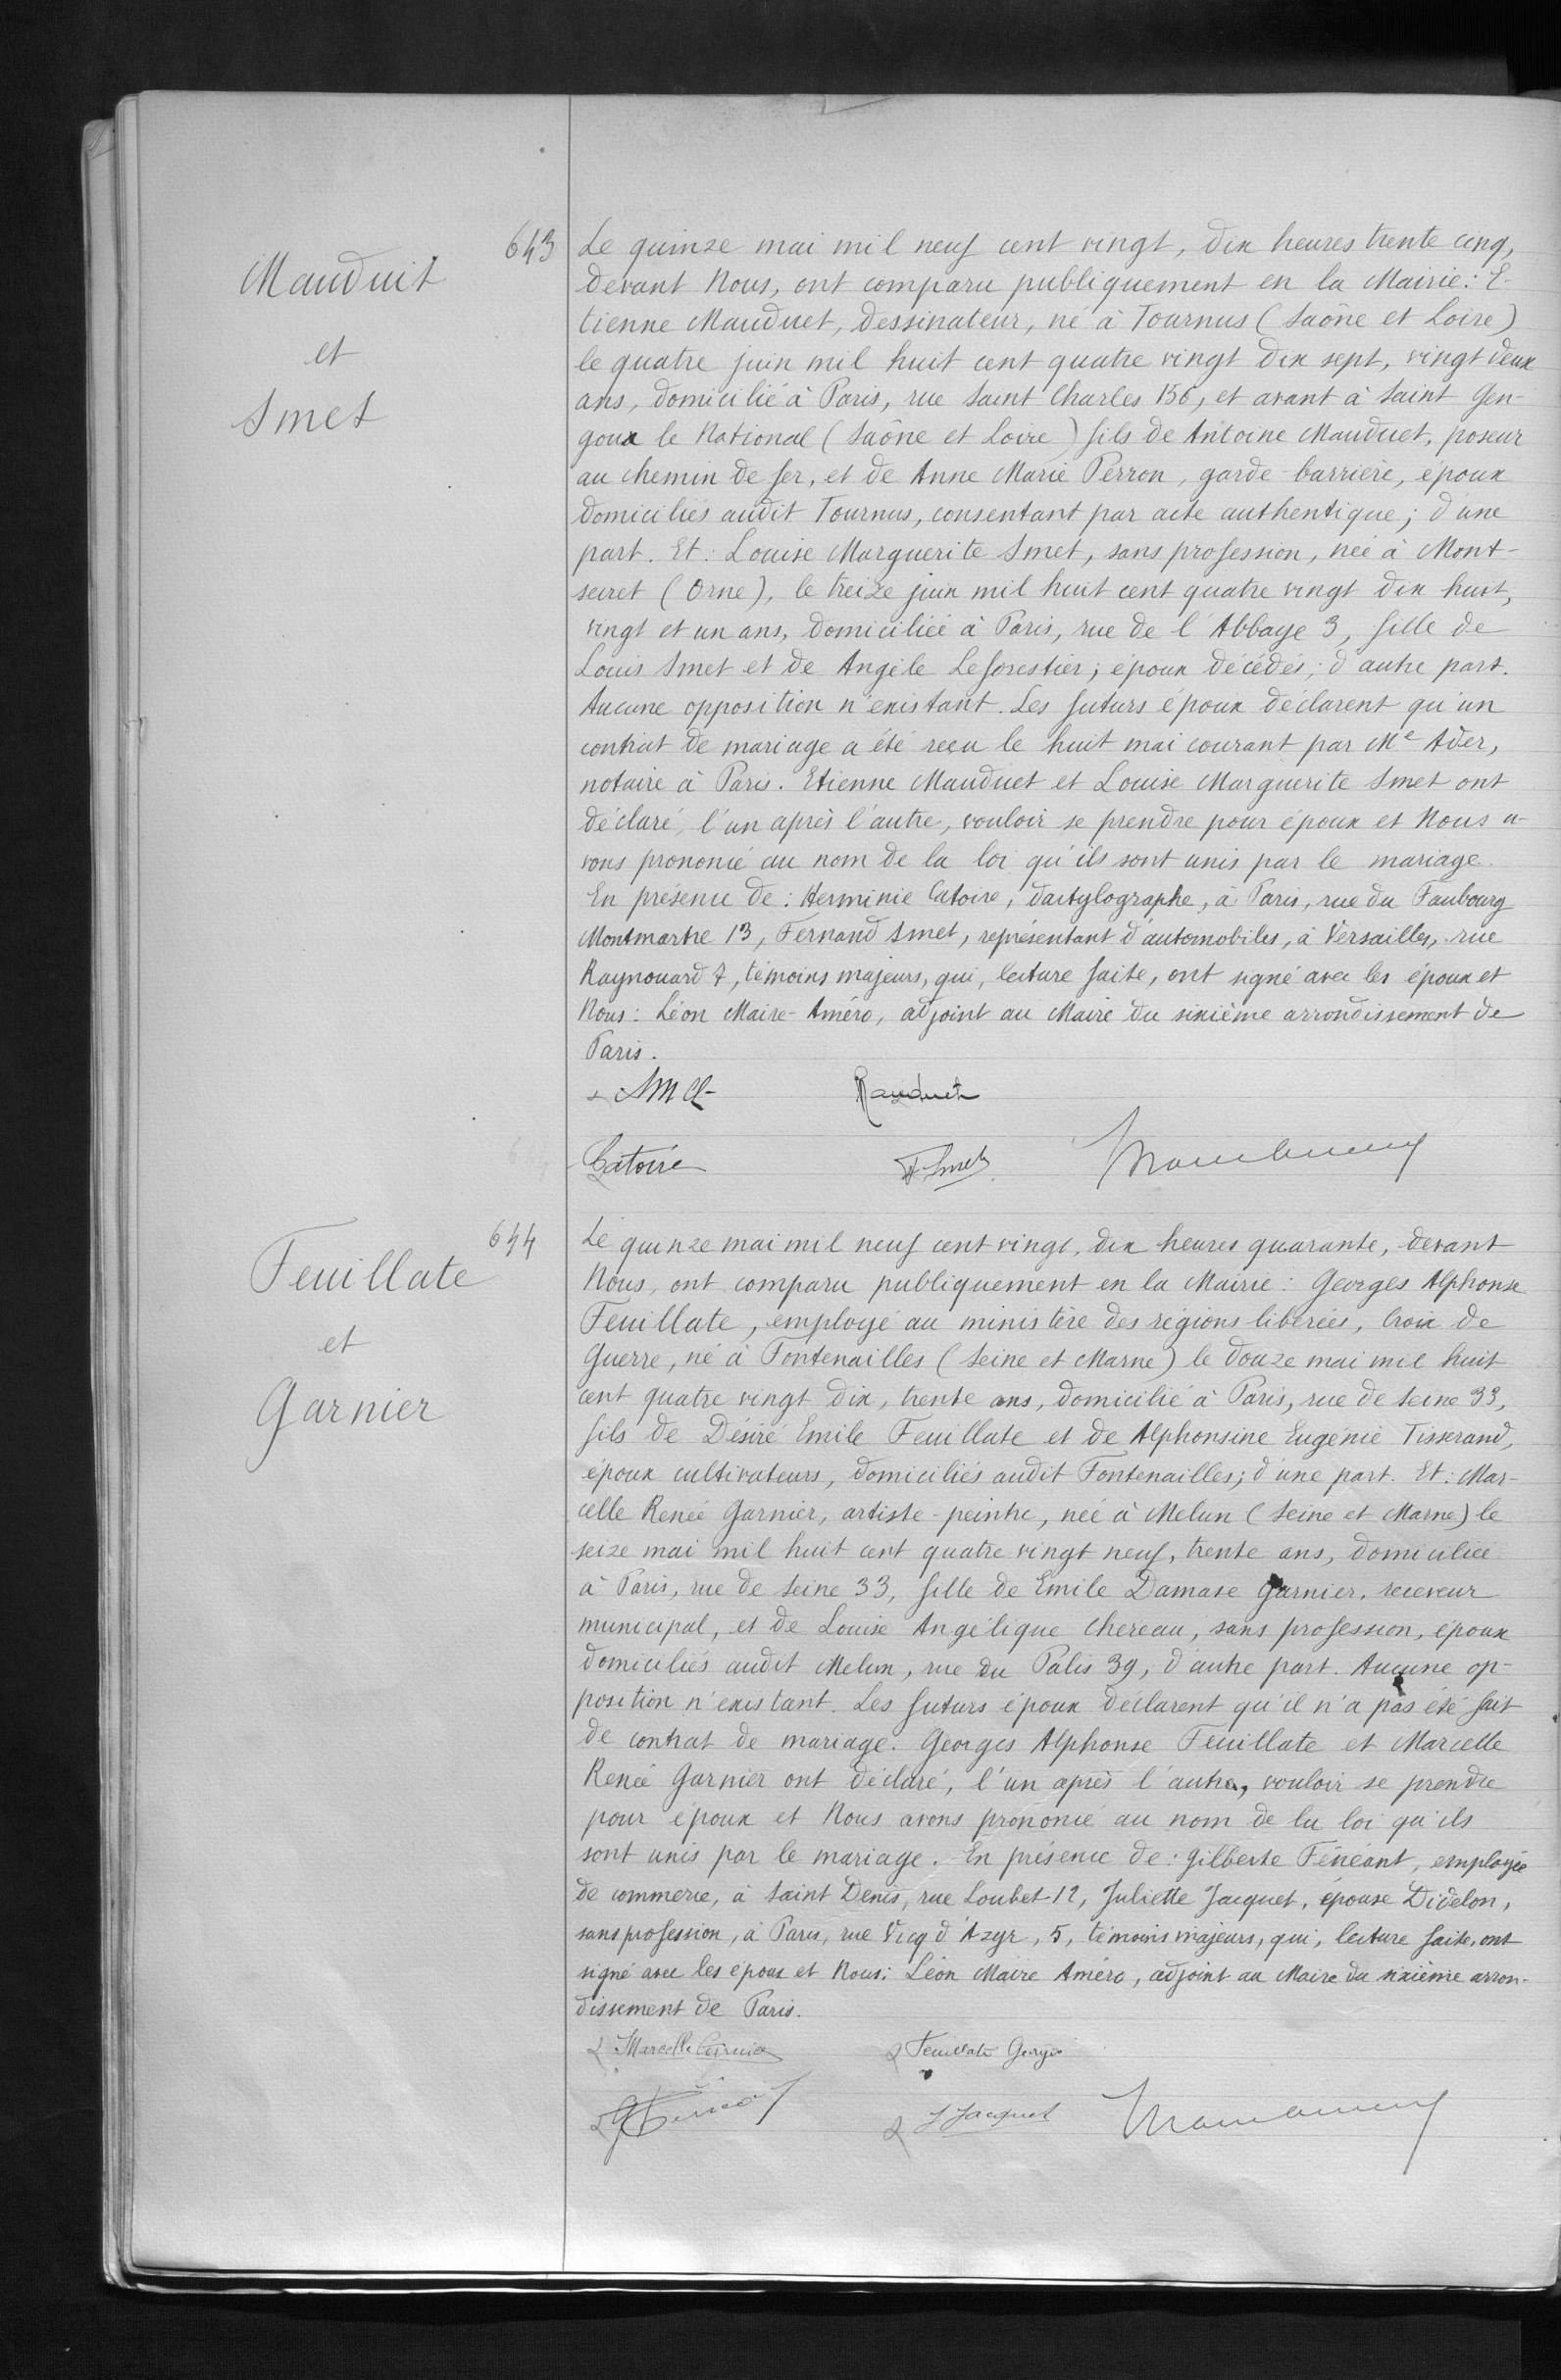643
Mauduit
et
Smes
Le quinze mai mil neuf cent vingt, dix heures trente cinq,
devant Nous, ont comparu publiquement en la Mairie: E
tienne Mauduit, dessinateur, né à Tournus (Saône et Loire)
le quatre juin mil huit cent quatre vingt dix sept, vingt deux
ans, domicilié à Pais, rue Saint Charles 156, et avant à Saint Gen-
goux le National (Saône et Loire) fils de Antoine Mauduit, poseur
au chemin de fer, et de Anne Marie Perro, garde-barrière, époux
domiciliés audit Tournus, consentant par acte authentique; d'une
part. Et: Louise Marguerite Smes, sans profession, née à Mont-
seiret (Orne), le treize juin mil huit cent quatre vingt dix huit,
vingt et un ans, domiciliée à Paris, rue de l'Abbaye 3, fille de
Louis Amet et de Angèle Leforestier, époux décédés; d'autre part.
Aucune opposition n'existant. Les futurs époux déclarent qu'un
contrat de mariage a été reçu le huit mai courant par Me Ader,
notaire à Paris. Etienne Mauduit et Louise Marguerite Smes ont
déclaré l'un après l'autre vouloir se prendre pour époux et Nous a-
vons prononcé au nom de la loi qu'ils sont unis par le mariage.
En présence de: Herminie Caloire, dactylographe, à Paris, rue du Faubourg
Montmartre 13, Fernand Smes, représentant d'automobile, à Versailles, rue
Raymouard 7, témoins majeurs, qui, lecture faite, ont signé avec les époux et
Nous: Léon Maire-Améro, adjoint au Maire du sixième arrondissement de
Paris.
644
Feuillate
et
Garnier
Le quinze mai mil neuf cent vingt, dix heures quarante, devant
Nous, ont comparu publiquement en la Mairie: Georges Alphonse
Feuillate, employé au ministère des régions libérées, Croix de
Guerre, né à Fontenailles (Seine et Marne) le douze mai mil huit
cent quatre vingt dix, trente an, domicilié à Paris, rue de Seine 33,
fils de Désiré Emile Feuillate et de Alphonsine Eugénie Tisserand,
époux cultivateurs, domiciliés audit Fontenailles; d'une part. Et: Mar-
celle Renée Garnier, artiste-peintre, née à Melun (Seine et Marne) le
seize mai mil huit cent quatre vingt neuf, trente ans, domiciliée
à Paris, rue de Seine 33, fille de Emile Damade Garnier, receveur
municipal, et de Louise Angélique Chereau, sans profession, époux
domiciliés audit Melun, rue du Palis 39, d'autre part. Aucune op-
position n'existant. Les futurs époux déclarent qu'il n'a pas été fait
de contrat de mariage. Georges Alphonse Feuillate et Marcelle
Renée Garnier ont déclaré, l'un après l'autre, vouloir se prendre
pour époux et Nous avons prononcé au nom de la loi qu'ils
sont unis par le mariage. En présence de: Gilberte Fénéant, employée
de commerce, à Saint Denis, rue Loubet 12, Juliette Jacquet, épouse Didelon,
sans profession à Paris, rue Vicq d'Azyr, 5, témoins majeurs, qui, lecture faite, ont
signé avec les époux et Nous: Léon Maire Améro, adjoint au Maire du sixième arron-
dissement de Paris.
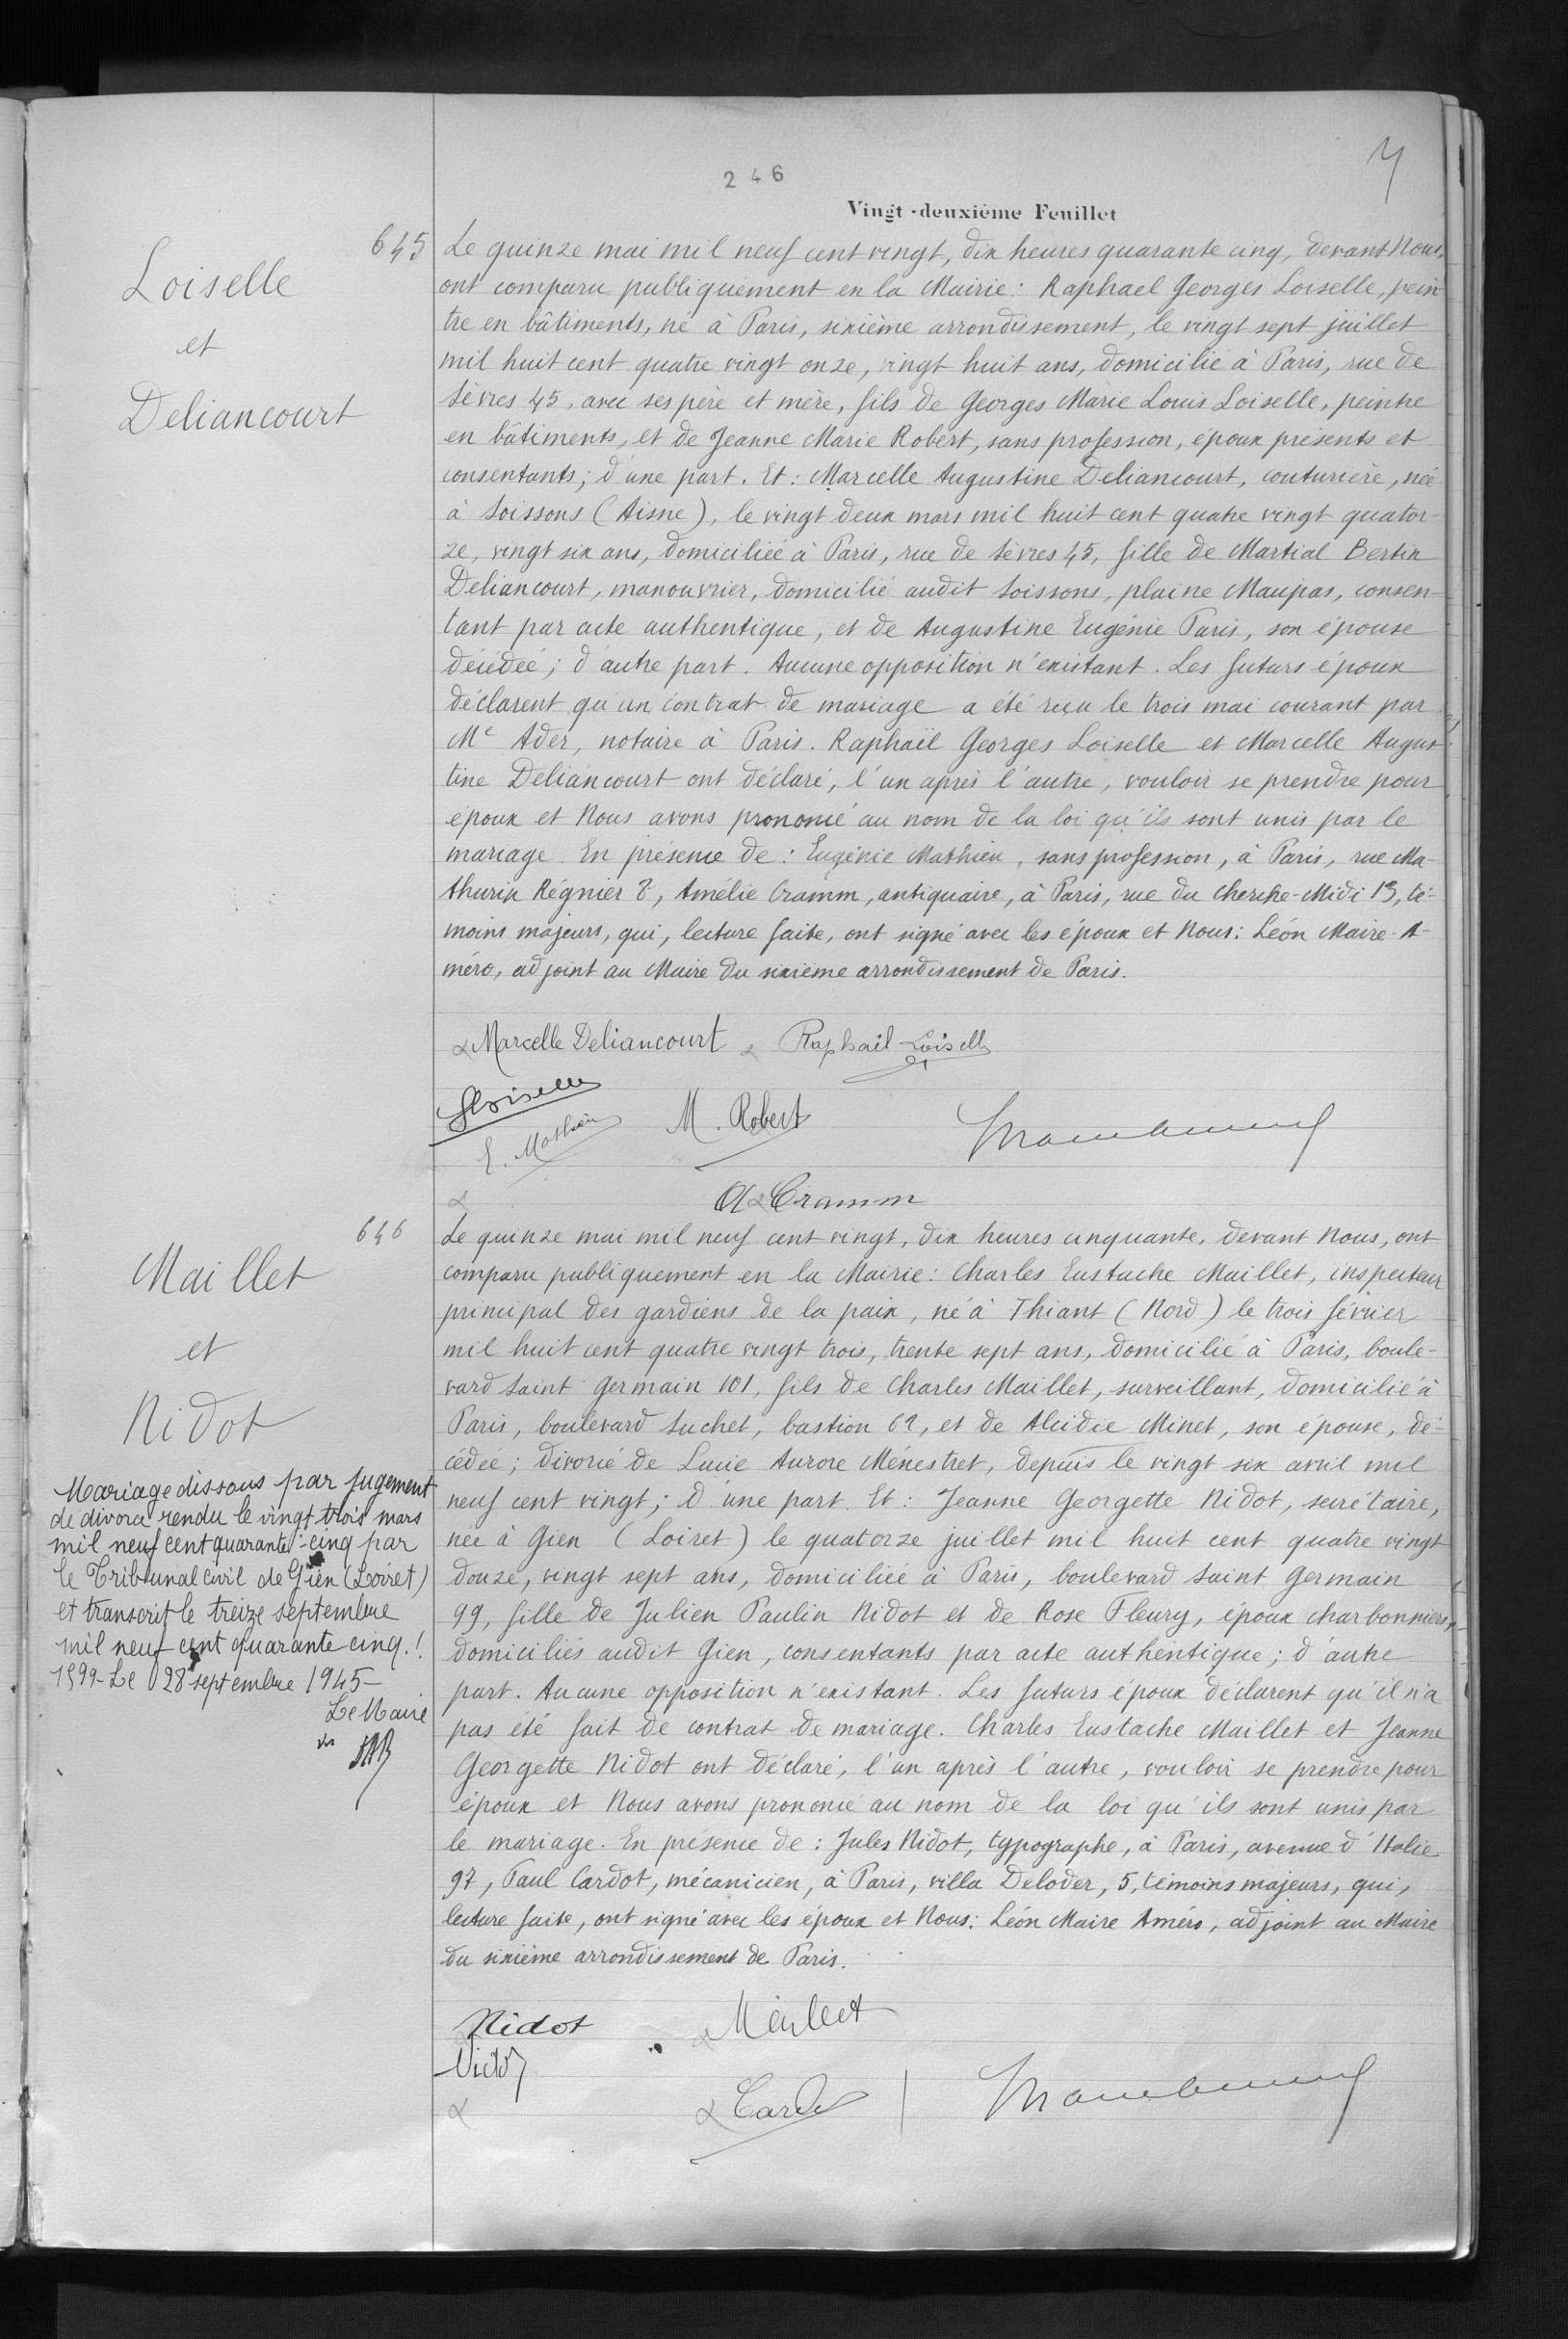645
Loiselle
et
Deliancourt
Le quinze mai mil neuf cent vingt, dix heures quarante cinq, devant Nous,
ont comparu publiquement en la Mairie: Raphael Georges Loiselle, pein-
tre en bâtiments, né à Paris, sixième arrondissement, le vingt sept juillet
mil huit cent quatre vingt onze, vingt-huit ans, domicilié à Paris, rue de
Sèvres 45, avec ses père et mère, fils de Georges Marie Louise Loiselle, peintre
en bâtiments, et de Jeanne Marie Robert sans profession, époux présents et
consentants; d'une part. Et: Marcelle Augustine Déliancourt, couturière, née
à Soissons (Aisne), e vingt deux mars mil huit cent quatre vingt quator-
ze, vingt six ans, domiciliée à Paris, rue de Sèvres 45, fille de Martial Bertin
Deliancourt, manouvrier, domicilié audit Soissons, plaine Maupas, consen-
tant par acte authentique, et de Augustine Eugénie Paris, son épouse
décédée; d'autre part. Aucune opposition n'existant. Les futurs époux
déclarent qu'un contrat de mariage a été reçu le trois mai courant par
Me Ader, notaire à Paris. Raphaël Georges Loiselle et Marcelle Augus
tine Deliancourt ont déclaré, l'un après l'autre, vouloir se prendre pour
époux et Nous avons prononcé au nom de la Loi qu'ils sont unis par le
mariage. En présence de: Eugénie Mathieu, sans profession, à Paris, rue Ma-
thurin Régnier 8, Amélie Cramm, antiquaire, à Paris, rue du Cherche-Midi 13, té-
moins majeurs, qui, lecture faite, ont signé avec les époux et Nous: Léon Maire A-
méro, adjoint au Maire du sixième arrondissement de Paris.
646
Maillet
et
Nidot
Le quinze mai mil neuf cent vingt, dix heures cinquante, devant Nous, ont
comparu publiquement en la Mairie: Charles Eustache Maillet, inspecteur
principal des gardiens de la paix, né à Thiant (Nord) le trois février
mil huit cent quatre vingt trois, trente sept ans, domicilié à Paris, boule-
vard Saint Germain 101, fils de Charles Maillet, surveillant, domicilié à
Paris, boulevard Suchet, bastion 61, et de Alcidie Minet, son épouse, dé-
cédée; divorcée de Lucie Aurore Ménestret, depuis le vingt-six avril mil
neuf cent vingt; d'une part. Et: Jeanne Georgette Nidot, secrétaire,
née à Gien (Loiret) le quatorze juillet mil huit cent quatre vingt
douze, vingt sept ans, domiciliée à Paris, boulevard saint germain
99, fille de Julien Paulin Nidot et de Rose Fleury, époux charbonniers,
domiciliés audit Gien, consentants par acte authentique; d'autre
part. Aucune opposition n'existant. Les futurs époux déclarent qu'il n'a
pas été fait de contrat de mariage. Charles Eustache Maillet et Jeanne
Georgette Nidot ont déclaré, l'un après l'autre, vouloir e prendre pour
époux et Nous avons prononcé au nom de la loi qu'ils sont unis par
le mariage. En présence de: Jules Nidot, typographe, à Paris, avenue d'Holie
97, Paul Cardot, mécanicien, à Paris, villa Deloder, 5, témoins majeurs, qui,
lecture faite, ont signé avec les époux et Nous: Léon Maire Améro, adjoint au Maire
du sixième arrondissement de Paris.